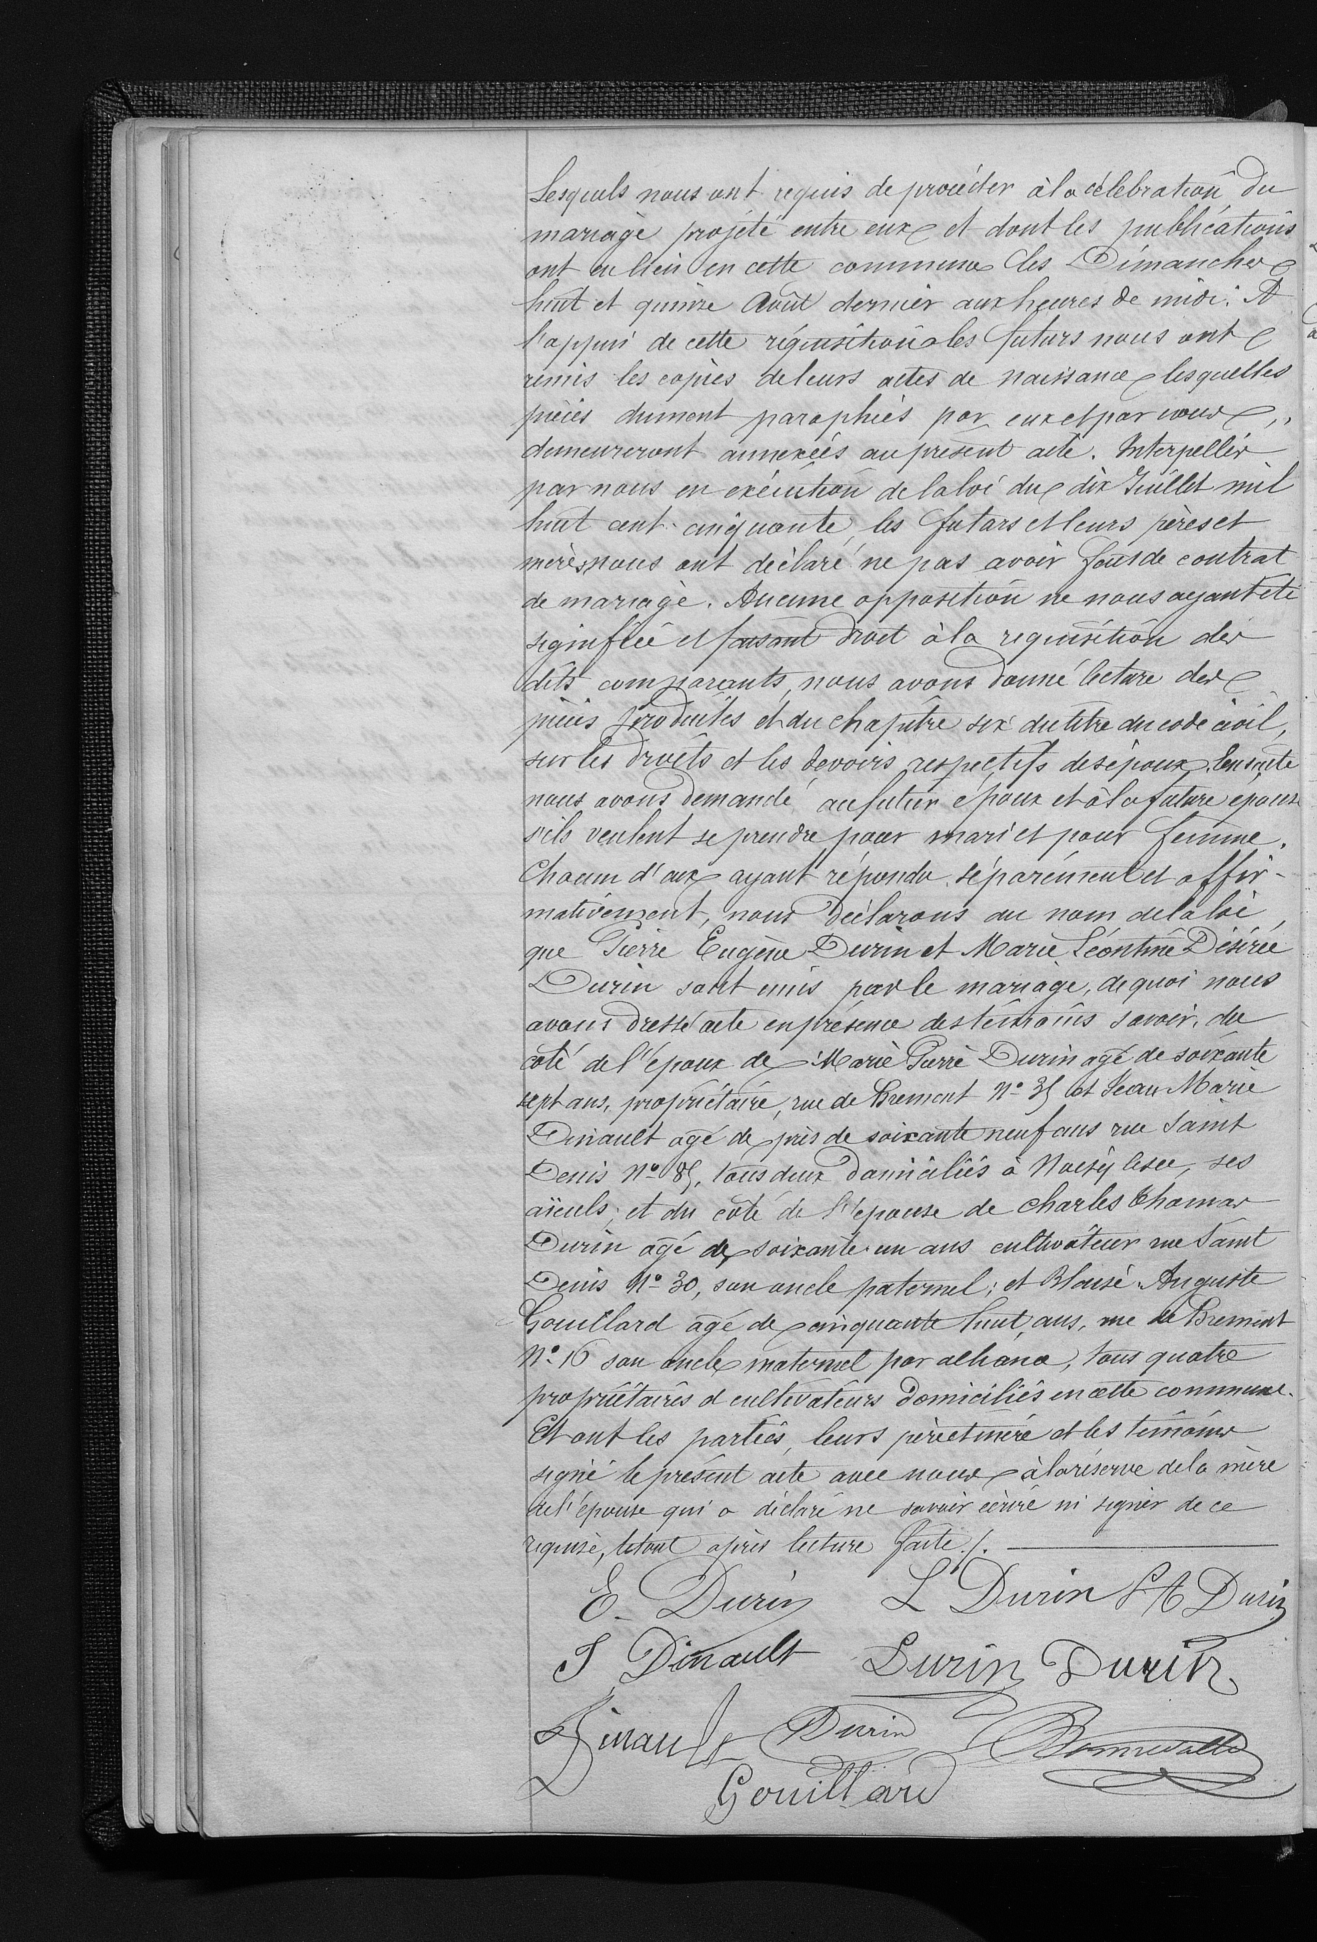Lesquels nous ont requis de procéder à la célebration du
mariage projeté entre eux et dont les publications
ont eu lieu en cette commune les Dimanches
huit et quinze août dernier aux heures de midi A
l'appui de cette réquisition les futurs nous ont
remis les copies de leurs actes de naissance lesquelles
pièces dument paraphées par eux et par nous
demeureront annexées au présent acte. Interpellés
par nous en execution de la loi du dix Juillet mil
huit cent cinquante, les futurs et leurs pères et
mères nous ont déclaré ne pas avoir fait de contrat
de mariage. Aucune opposition ne nous ayant été
signifiée et faisant droit à la réquisition des
dits comparants, nous avons donné lecture des
pièces produites et du chapitre six du titre du code civil,
sur les droits et les devoirs respectifs des époux, Ensuite
nous avons demandé au futur époux et à la future épouse
s'ils veulent se prendre pour mari et pour femme,
Chacun d'eux ayant répondu séparément et affir-
mativement, nous déclarons au nom de la loi
que Pierre Eugène Durin et Marie Léontine Désirée
Durin sont unis par le mariage, de quoi nous
avons dressé acte en présence des témoins savoir du
coté de l'époux de Marie Pierre Durin âgé de soixante
sept ans, propriétaire, rue de Brement N°35 et Léon Marie
Dinault âgé de près de soixante neuf ans rue Saint
Denis N°-85, tous deux domiciliés à Massy Lesu, ses
aïeuls, et du code de l'épouse de Charles Chamar
Durin âgé de soixante un ans cultivateur rue Saint
Denis N°-30, son oncle paternel: et Blaise Auguste
Gourtlard âgé de cinquante huit ans, rue de Bremont
N°-16 son oncle maternel par alliance, tous quatre
propriétaires et cultivateurs domiciliés en cette commune
Et ont les parties, leur père et mère et les témoins
signé le présent acte avec nous à la réserve de la mère
de l'épouse qui a déclaré ne savoir écrire ni signer de ce
requise, le tout après lecture faite ./.
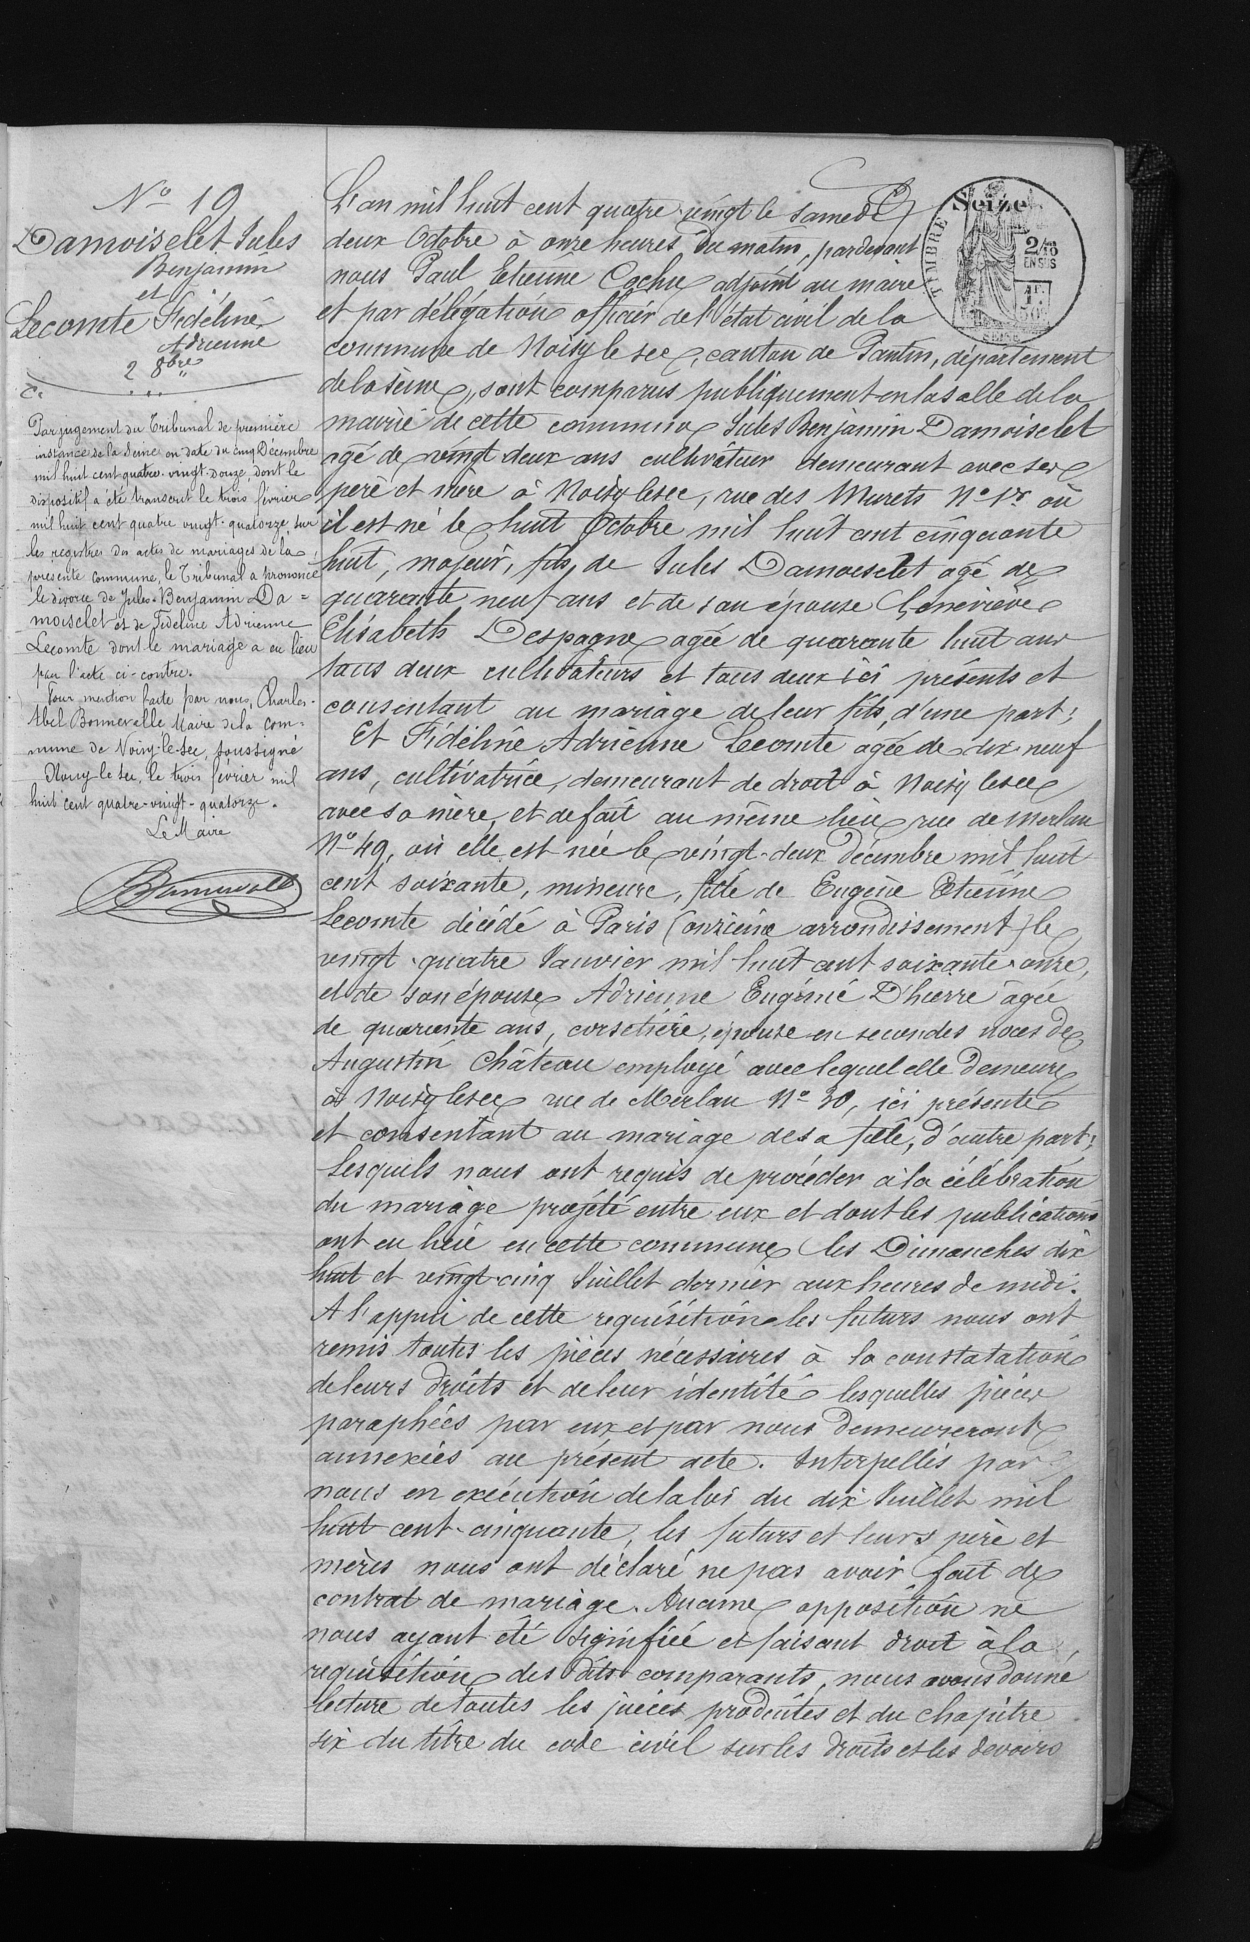N°-19
Damoiselet Jules
Benjamin
et
Lecomte Fidéline
Adrienne
28 bre
L'an mil huit cent quatre vingt le samedi
deux Octobre à onze heures du matin, pardevant
nous Paul Etienne Cochu adjoint au maire
et par délégation officier de l'état civil de la
commune de Noisy le sec, canton de Pantin, département
de la Seine, sont comparus publiquement en la salle de la
mairie de cette commune Jules Benjamin Damoiselet
âgé de vingt-deux ans cultivateur demeurant avec ses
père et mère à Noisy le sec, rue des Murets N°17 où
il est né le huit Octobre mil huit cent cinquante
huit, majeur, fils de Jules Damoiselet âgé de
quarante neuf ans et de son épouse Geneviève
Elisabeth Despagne agée de quarante huit ans
tous deux cultivateurs et tous deux ici présents et
consentant au mariage de leur fils, d'une part:
Et Fidéline Adrienne Lecomte agée de dix neuf
ans, cultivatrice, demeurant de droit à Noisy le sec
avec sa mère, et de fait au même lieu rue de Merlau
N°49 où elle est née le vingt deux décembre mil huit
cent soixante, mineure, fille de Eugène Etienne
Lecomte décédé à Paris (onzième arrondissement) le
vingt quatre Janvier mil huit cent soixante onze
et de son épouse Adrienne Eugénie Dhurre âgée
de quarante ans, corsetière, épouse en secondes noces de
Augustin Château employé avec lequel elle demeure
à Noisy le sec rue de Merlau N°30, ici présents
et consentants au mariage de sa fille, d'autre part:
Lesquels nous ont requis de procéder à la célébration
du mariage projeté entre eux et dont les publications
ont eu lieu en cette commune les Dimanches dix
huit et vingt-cinq Juillet dernier aux heures de midi:
A l'appui de cette requisition les futurs nous ont
remis toutes les pièces nécessaires à la constatation
de leurs droits et de leur identité lesquelles pièces
paraphées par eux et par nous demeureront
annexées au présent acte. Interpellés par
nous en exécution de la loi du dix Juillet mil
huit cent-cinquante les futurs et leurs père et
mères nous ont déclaré ne pas avoir fait de
contrat de mariage. Aucune opposition ne
nous ayant été signifiée et faisant droit à la
requisition des faits comparants, nous avons donné
lecture de toutes les pièces produites et du chapitre
six du titre du code civil sur les droits et les devoirs